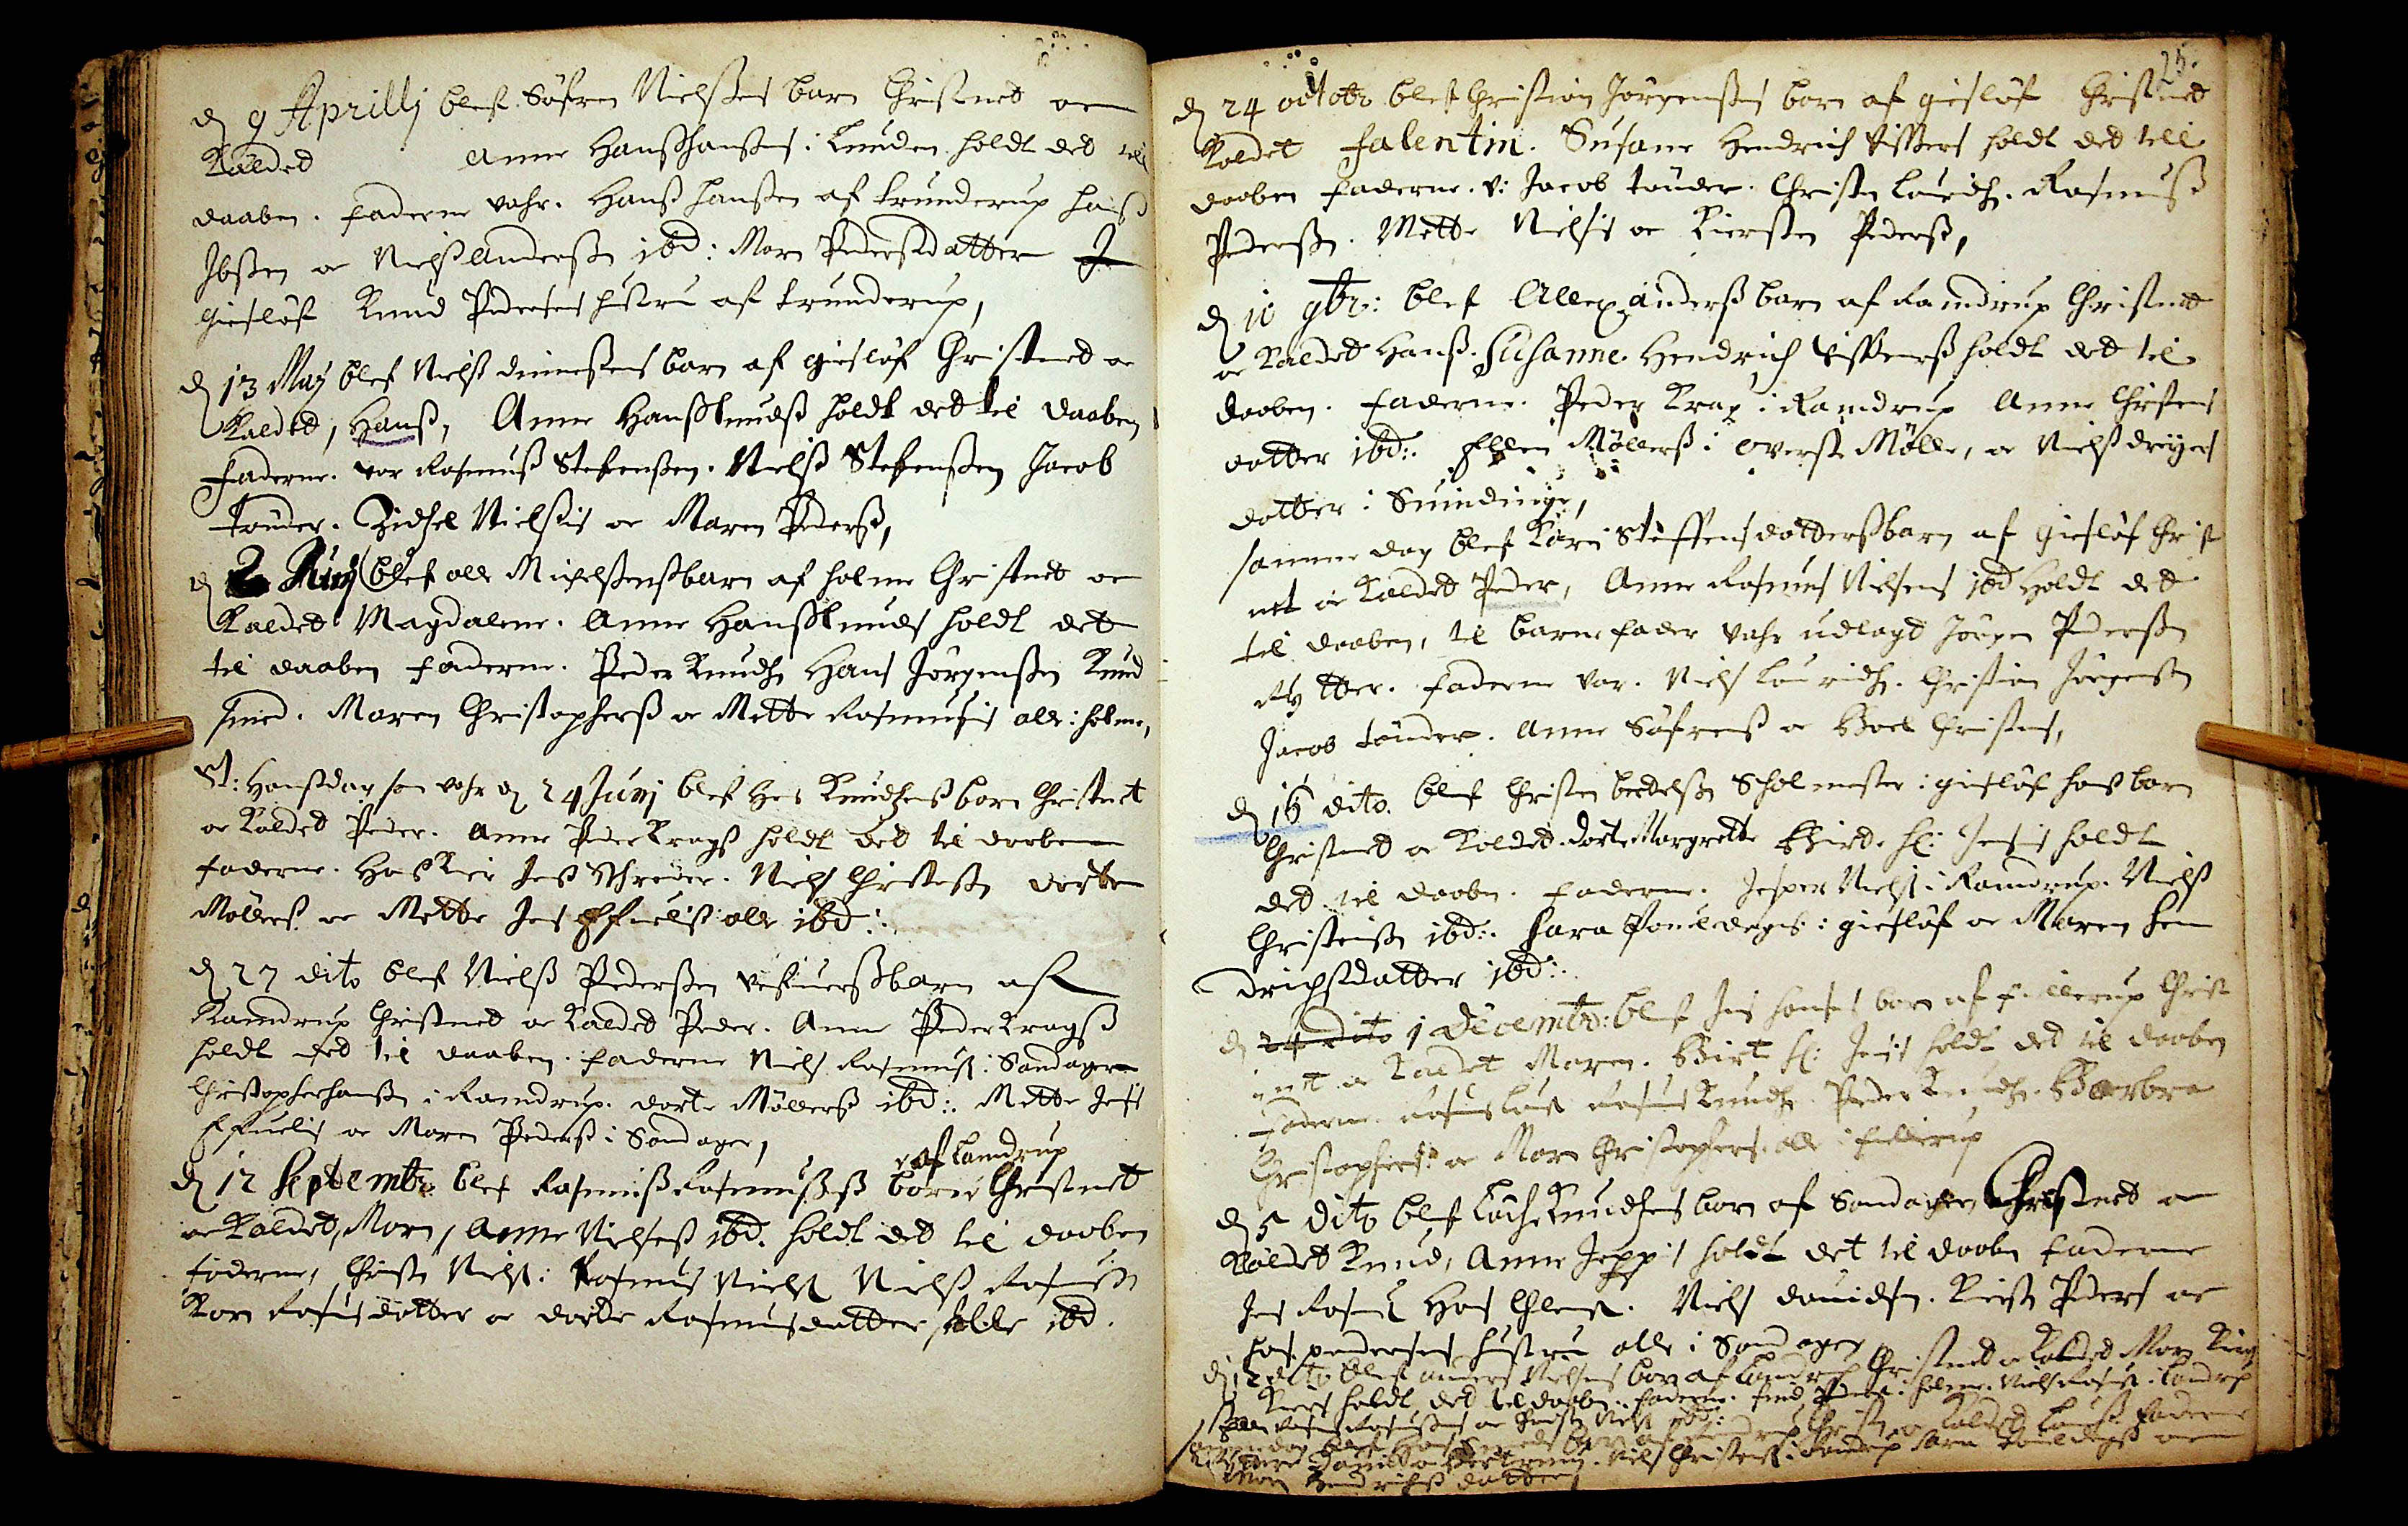d 9 Aprillij blef Søfren Nielssens barn Christenet oc
kaldet
Anne Hans Hansens i Lunden, holdt det til
daaben. Faderne vahr. Hans Hansen af Trunderup Hanss
Ibsen oc Niels Andersen ibd: Maren Pedersdatter I
Giesløf Knud Pedersens hustru af Trunderup,
d 13 Maij blef Niels Dinnessens barn af Giesløff Christnet oc
kaldet, Hanss, Anne Hans Knudss holdt det til Daaben
Faderne. var Rasmuss Stefenssen. Nielss Stefenssen Jacob
Tønder. Zidtzel Nielssis oc Maren Pederss,
d 2 Junij blef olle Michelssens barn af holme Christnet oc
kaldet Magdalene. Anne Hans Knuds holdt det
til daaben Faderne. Peder Knuds Hans Jørgenssen Knud
smed. Maren Christopherss oc Mette Rasmusis alle i holme,
St: Hanssdag som vahr d 24 Junij blef Hes Knudzenss barn Christnet
oc kaldet Peder. Anne Peder Ktragss holdt det til daaben
Faderne H## Jess Schreder. Niels Chrstensen Dorte
Møllers oc Mette Jens ##fuels alle ibd:.
d 27 dito blef Nielss Pederssen Vefuers barn af
Ramdrup christnet oc kaldet Peder. Anne Peder Kragss
holdt det til daaben. Faderne Niels Rasmuss i Sandager
Ciestopher Hanssen i Ramdrup. dorte Møllers ibd:. Mette Jensis
Efuelis oc Maren Peders i Sandager,
v aff Landrup
d 12 Septembr. blef Rasmuss Rasmussenss barn v Christnet
oc kaldet, Marn, Anne Nielses ibd. holdt det til daaben
Faderne, Christe Niels: Rasmus Niels Niels Rasmuss
Kore Rasmusdatter oc dorde Rasmusdatter alle ibd.
25.
d 24 Octobr blef Christian Jørgensn barn af giesløf Christnet
kaldet Falentin. Susane Hendrich Vistters holdt det till
daaben Faderne. v: Jacob tønder. Christen Laudts. Rasmuss
Pederss Mette Nielsis oc Kiersten Pederss,
d 10 9br: blef Allex Anderss barn af Ramdrup Christinet
oc kaldet Hanss. Susanne Hendrich Vistters holdt det til
daaen. Faderne. Peder Krag i Ramdrup Anne Christens
datter ibd:. Ellen Møllerss i overst Mølle, oc Niels Dreyers
datter i Suindinge,
samme dag blef Karn Steffensdatters barn af Giesløf Christ
net oc kaldet Peder, Anne Rasmus Nielsens ibd Holdt det
til daaben, til barnefader vahr vdlagt Jørgen Pedersen
Rytter. Faderne var. Niels Laurids, Christen Jørgensen
Jacob tønder. Anne Søfrens oc Boel Christens,
d 16 dito. blef Christen Bertelss Scholemester i Giesløf hans barn
Christnet oc kaldet Dorte Margrette Birde hl: Jensis holdt
det til daabn. Faderen Jesper Niels i Ramdrup. Niels
Christensen ibd: Sara Pouel degns i giesløf oc Maren Hen¬
drichsdatter ibd:
d 24 dito 1 Decembr: blef Jens Hanses barn af Fiellerup Christ
= nett oc taldet Maren. Birte hl: Jens holdt det til daaben
Faderne Rasmus Laus  Rasmus Kunds Peder Knuds Barbra
Christophers oc Maren Christophers i Fiellerup
d 5 dito blef Laus Kudsens barn af Sandager christnet oc
kaldet Knud, Anne Jeppis holdt det til daabn faderne
Jep Rasmus Hans Chlemens. Niels Dauidsen. Kirsten Peders oc
Jens pedersens hustru alle i Sandager
d 12 dito blef Anders Nielsens barn af Lamdrup Christnet oc kaldet  Maren K##
Kiers holdt det til daaben. Faderne Find Peders i holme Niels Rasmus i Lamdrup
## Rasmus Andersenss oc Ch## Ni## ibd:
sammedag blef Hans Smeds barn af Ramdrup christnet og kaldet Lauss Faderene
## Dauid oc ## Niels Christens i Ramdrup Sara Pouels degns oc
Karen Hendrichss datter,
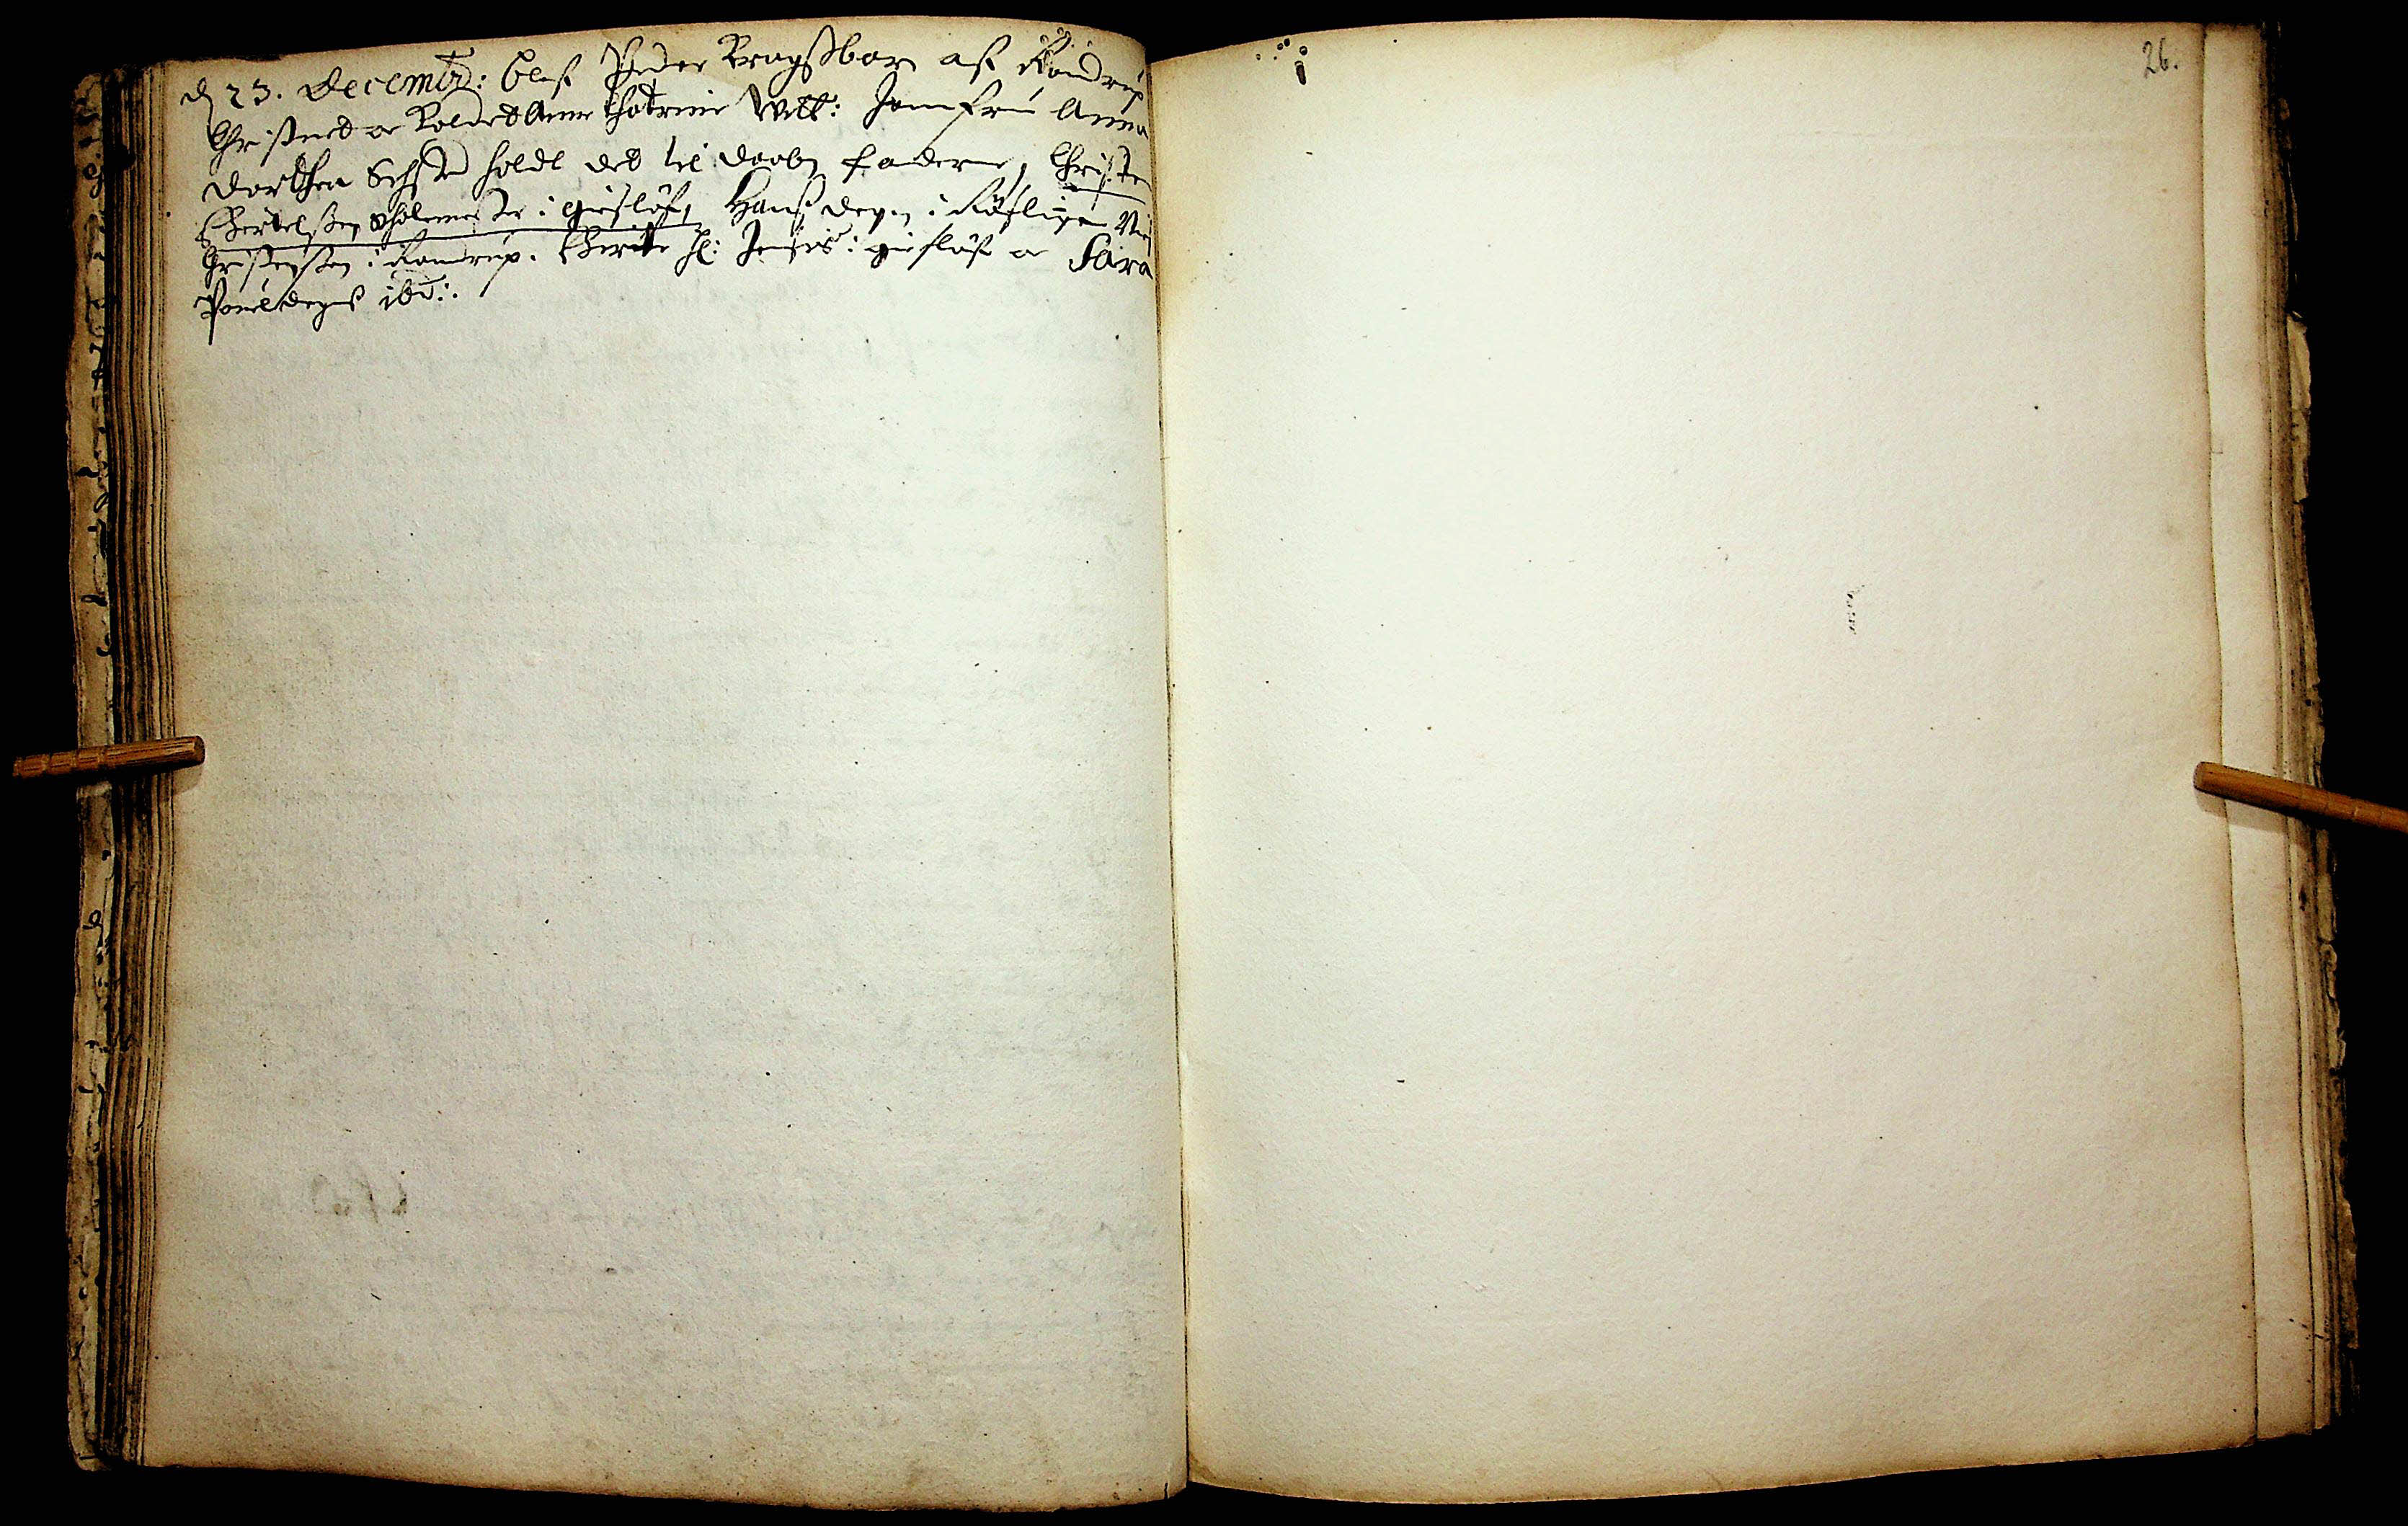d 23. Decembr: bleff Peder Krags barn af Ramdrup
Christnet oc kaldet Anne Chatrine Welb: Jomfru Anne
Dorthe Sehsted holdt det til daaben Faderne, Christen
Bertelsen Scholemester i Giesløf, Hanss dern i Røslige Ni##
Christenssen i Ramdrup. Birete Hl: Jensis i giesløf oc Sara
Pouel degns ibd:
26.